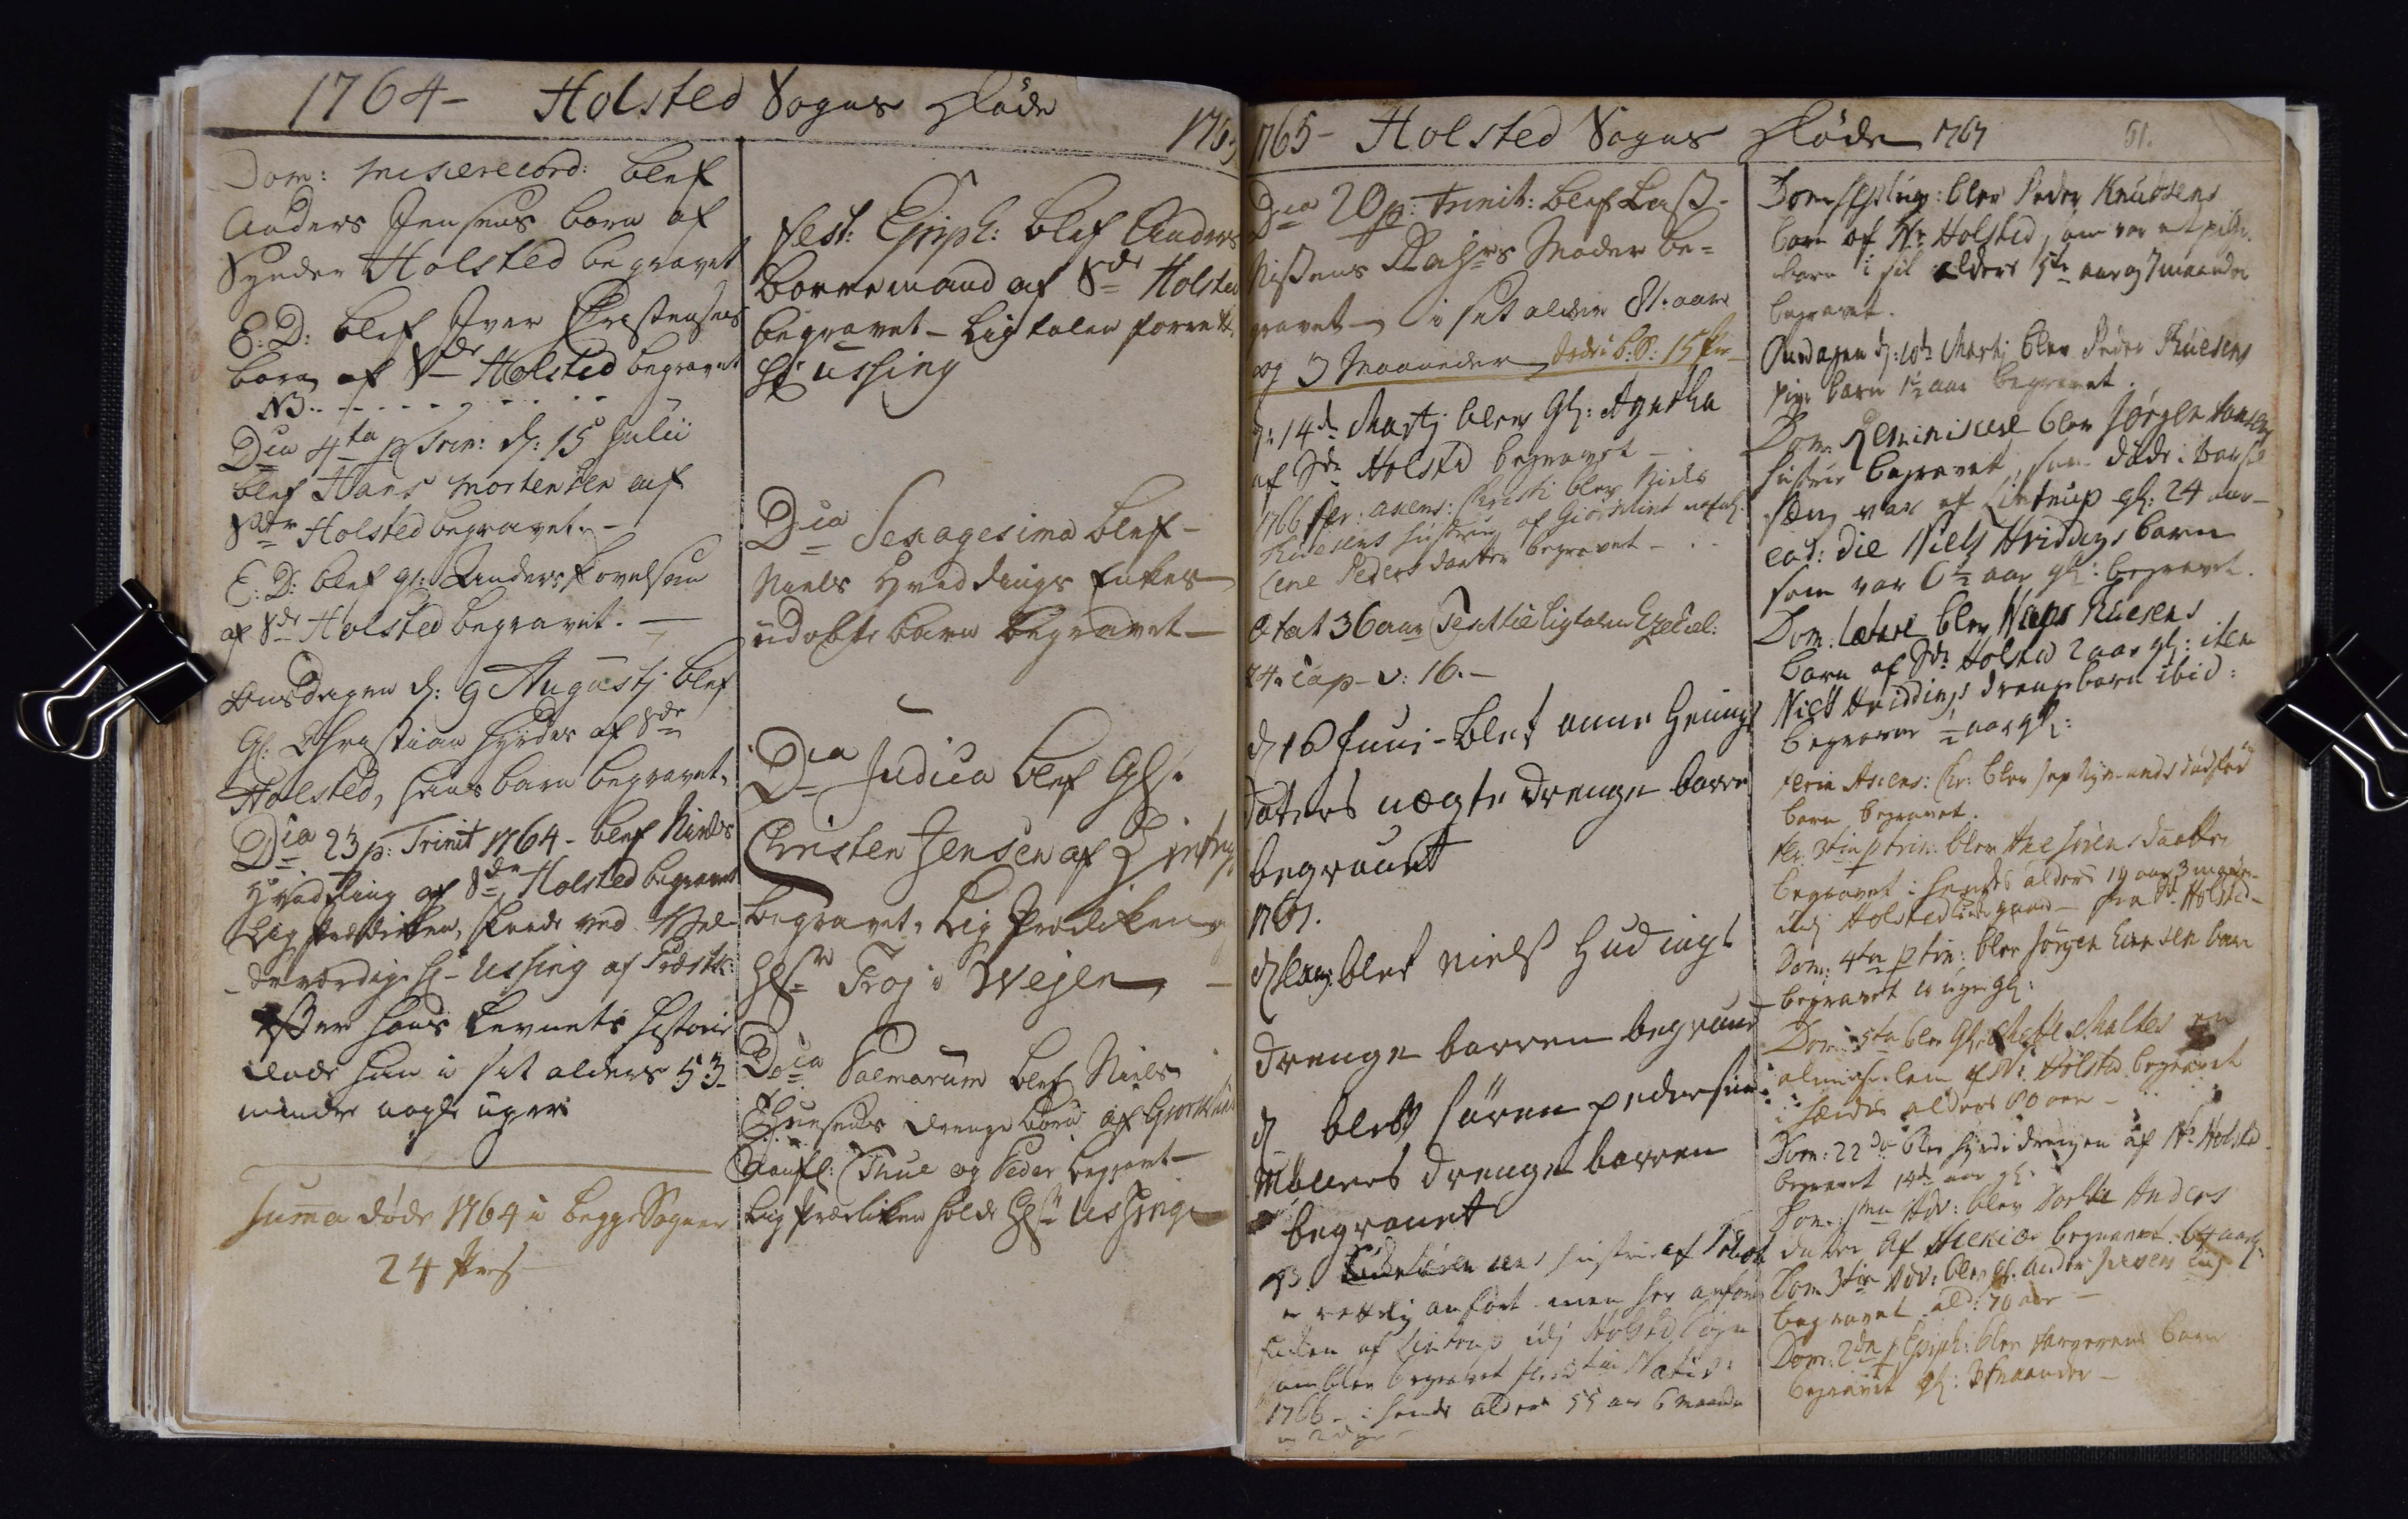1764.
Holsted Sogns Døde
Dom: Miserecord: blef
Anders Jensens barn af
Synder Holsted begravet
E: D: blef Iver Christensens
barn af Sdr Holsted begravet
NB
Dca 4ta p Trin: d: 15 Julii
blef Hans Mortensen af
Sdr Holsted begravet
E: D: blef gl: Anders Povelssen
af Sdr Holsted begravet.
Onsdagen d: 9 Augustj blef
gl: Christian Hyrdes af Sdr
Holsted, hans barn begravet,
Dca 23 p: Trinit 1764. blef Niels
Hvidding af Sdr Holsted begravet
Lig Prædiken, skeede ved Vel¬
erværdige Hl Ussing af Præstk:
## hans Levnets Historie
dode han i sit alders 53
mindre nogle uger.
suma døde 1764 i begge Sogne
24 Pers
1765
Fest: Epiph: blef Anders
Borremand af Sdr Holsted
begravet. - Lig talen forrett 
Hl ussing
Dca Sexagesima blef
Niels Hviddings Enkes
udobte barn begravet -
Dca Judica bleff Gl:
Christen Jensen af Lintrup
begravet, Lig Prædicken
Hlr Fog i Wejen
Doca Palmarum blef Niels
Thuesens Drenge børn af Giorklint
Nanfl: Thue og Peder begravet -
Lig Prædiken holde Hlr Ussing
1765 Holsted Sogns
Dca 20 p: Trinit: blef Lass
Nissens Kahrs Moder be¬
gravet i sit alders 81. aar.
og 3 Maaneder døde i b:S: 15 P##
d: 14de Martij blev gl: Antha
af Sdr Holsted begravet
1766 Fer: Anens: Christi blev Niels
Thuesens Hustrue, af Giørklint nafnl
Lene Peders daatter begravet -
ætat 36 Aar Tesct til Ligtalen Ezeciel:
24## cap - v: 16. -
d 16 Juni blef Anne Henngs
Datters uægte Drenge barn
begravet
1767
d Sexag: blef Niels Hudings
Drenge barren begruvet
d bleff søren pedersen
Møllers drenge barren
begravet
NB  ## hustru af Tobol
er rettelig anført men her anfo##
##den af Lintrup udj Holsted Sogn
som blev begravet ## Nativ:
1766 i hendes alders 55 aar 6 Maaneder
og 2 dage
51.
Døde 1767.
Dom Septug: blev Peder Knudsens
Barn af Nr Holsted, som var et Pige
barn i sit alders 5te aar og 7 maander
begravet
Onsdagen d: 10de Martj blev Peder Tuesens
pige barn 1½ aar begravet.
Dom: Reminiscere blev Jørgen Hansen
hustrue begravet, som døde i barsel
sæng var af Lintrup gl: 24 aar -
eod: die Niels Hviddings barn
som var 6½ aar gl: begravet
Dom: Lætare blev Hans Thuesens
barn af Sdr Holsted 2 Aar gl: item
Niels Hviddings drengebarn ibid
begravne ½ aar gl:
Feria Ascens: Chr: blev Jep Nymands dødfød
barn begravet
Fer: 3tia Trin: blev Ane Sørens daatter
begravet hendes alders 14 aar 3 maaneder
udj Holsted Kiærgaard - fra Sdr Holsted -
Dom: 4ta p Trin: blev Jørgen Larsen barn
begravet 10 uger gl
Dom: 5ta ble, Gl: Mette Maltes en
almisselem af Nr Holsted begravet
i hændes alders 80 aar
Dom: 22de blev hyrde drengen af Nr Holsted
begravet 14de aar gl:
Dom: 1ma Adv: blev Dorthe Anders
datter af Hilkiær begravet 64 aar gl:
Dom 3tia Nov. blev gl. Anders Jensens liig
begravet ald: 70 aar
Dom: 2da Epiph: blev farverens barn
begravet gl: 3 maaneder -
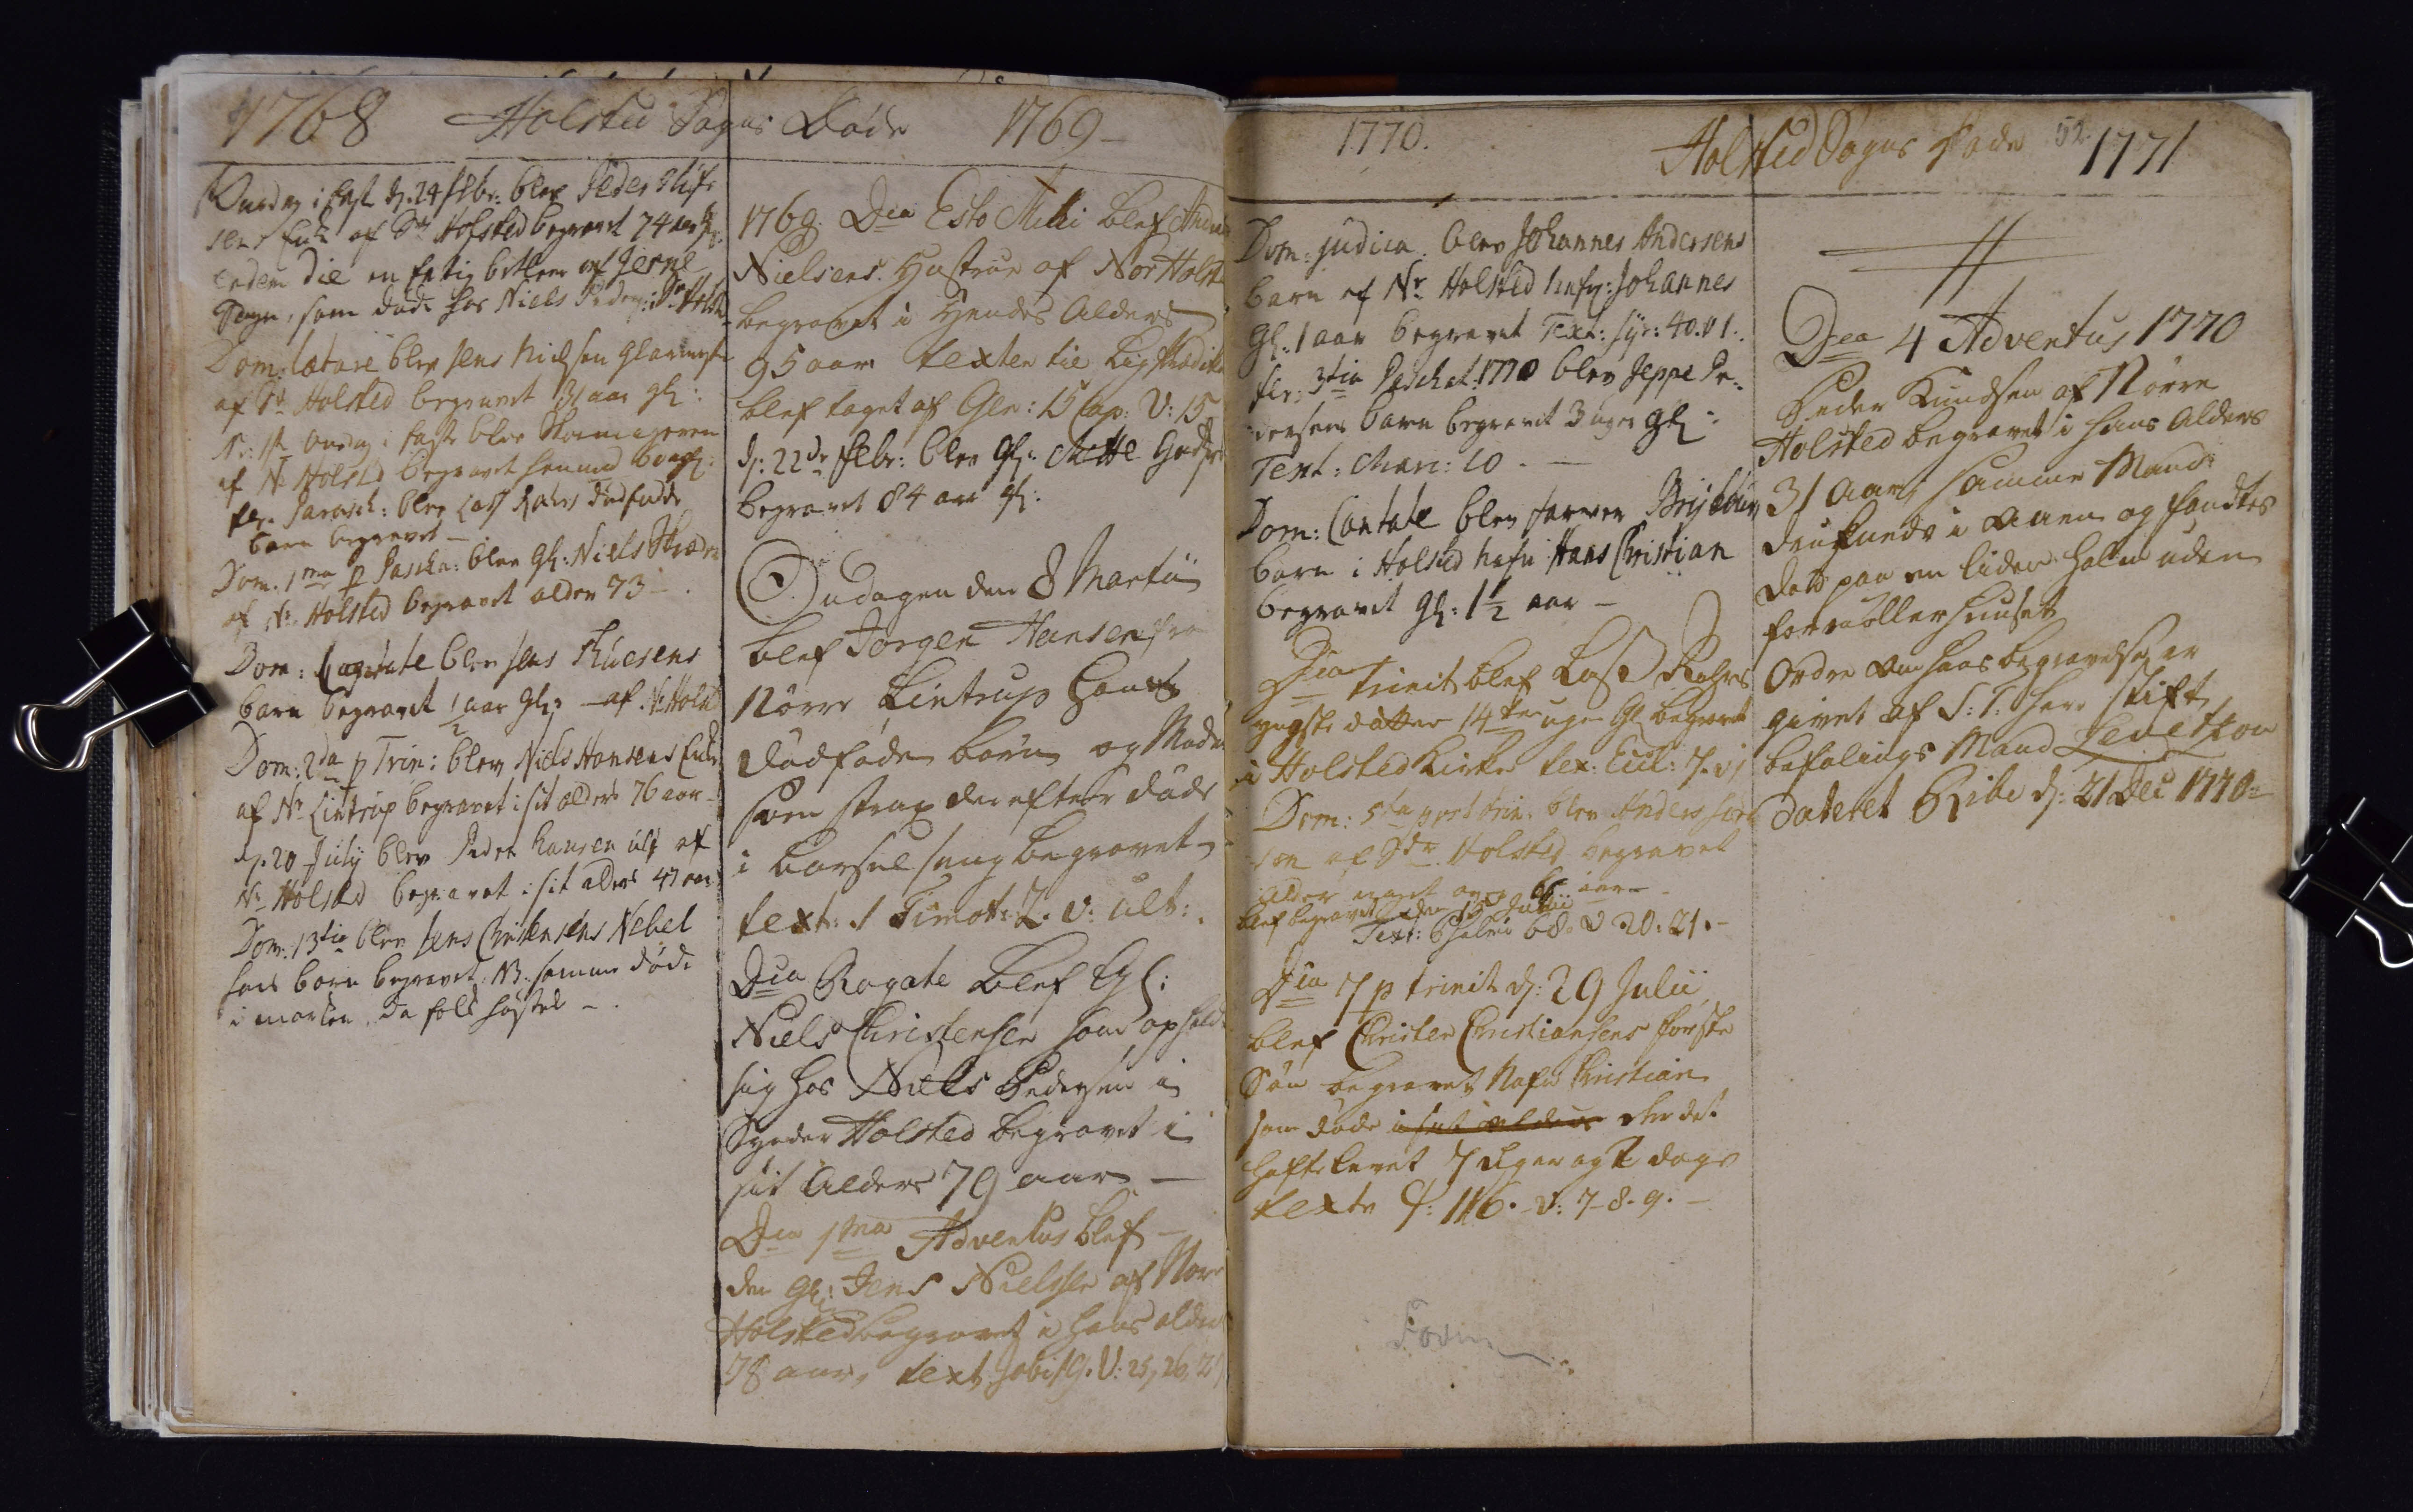Holsted Sogns Døde
1768
Onsdag i Faste d: 24 febr: blev Peder ##
sens af Enke af Sdr Holsted begravet 74 aar gl:
Eodem Die en fattig betler af Jerne
Sogn, som døde hos Niels Peders: i Sdr Holsted
Dom: Lætare blev Jens Nielssen Glarmester
af Sdr Holsted begravet 31 aar gl:
## 1st onsdag i faste blev Skoemageren
af N Holsted begravet henved 60 aar gl:
Fer. Parasch: blev Lass Rahns dødfodde
barn begravet
Dom: 1ma p Pascha: blev gl: Niels Skræder
af Nr Holsted begravet alder 73
Dom: Cantate blev Jens Tuesens
barn begravet ½ aar gl: - af Nr Holsted
Dom: 2da p Trin: blev Niels Hansens Enke
af Nr Lintrup begravet i sit alders 76 aar.
d: 20 Julij blev Peder Hansen ulf af
Nr Holsed begravet i sit alders 47 aar
Dom: 13tia blev Jens Christensens Nebel
hans barn begravet: NB: samme døde
i marken, da folk høstet -
1769
1769. Dca Esto Mihi blef Anders
Nielsens Hustrue af Nor Holsted
begravet i Hendes Alders
95 Aar texten til Lig Prædike
blef taget af Gle: 15 Cap: v: 15
d: 22de febr: blev gl: Mette G##
begravet 84 Aar gl:
Ondagen den 8 Martii
blef Jorgen Hansen fra
Nørre Lintrup Hans
dødføde barn og Moder
som strax derefter døde
i Barselseng Begravet.
Text: 1 Timot: 2. v: ult:
Dca Rogate blev Gl:
Niels Christensen som ophold 
sig hos Niels Pedersen i
Syder Holsted begravet i
sit Alders 79 Aar.
Dca 1ma Adventus blef
den gl: Jens Nielsen af Nor
Holsted begravet i hans alder
78 aar. text Jabit## v: 25, 26, 27
1770.
Holsted Sogns Dode
Dom: Judica blev Johannes Andersens
barn af Nr Holsted nafn: Johannes
gl: 1 Aar begravet Text: fyr: 40.01
Fer: 3tia Paschat: 1770 blev Jeppe Pe¬
dersens barn begravet 3 uger gl:
Text: Man: 10.
Dom: Cantate blev farver Bryllus
barn i Holsted Nafn Hans Christian
begravet gl: 1½ aar -
Dca Trinit blef Lass Rahrs
yngste datter 14ten uger gl. begravet
i Holsted Kirke tex: Eul: 7. v 1
Dom: 5ta post Trin: blev Anders ##
sen af Sdr Holsted begravet
alder noget over 66 aarr
blef begravet den 15 Junii
Text: Phalm 68 v 20: 21.
Dca 7 p Trinit d: 29 Julii
blef Christen Christiansens første
Søn begravet Nafn Christian
som døde i sit alders der det
hafte levet 7 uger og 2 dage.
texte ##: 116. v: 7-8-9.
52.
1771.
Dca 4 Adventus 1770
Peder Knudsen af Nørre
Holsted begravet i hans Alders
31 Aar samme Mand
druknede i Aaen og fandtes
dod paa en liden Holm uden
for møller Huuset
Ordre om Hans begravelse er
givet af S: T: here stift
befalings Mand Levetzon
dateret Ribe d: 21 Dec: 1770